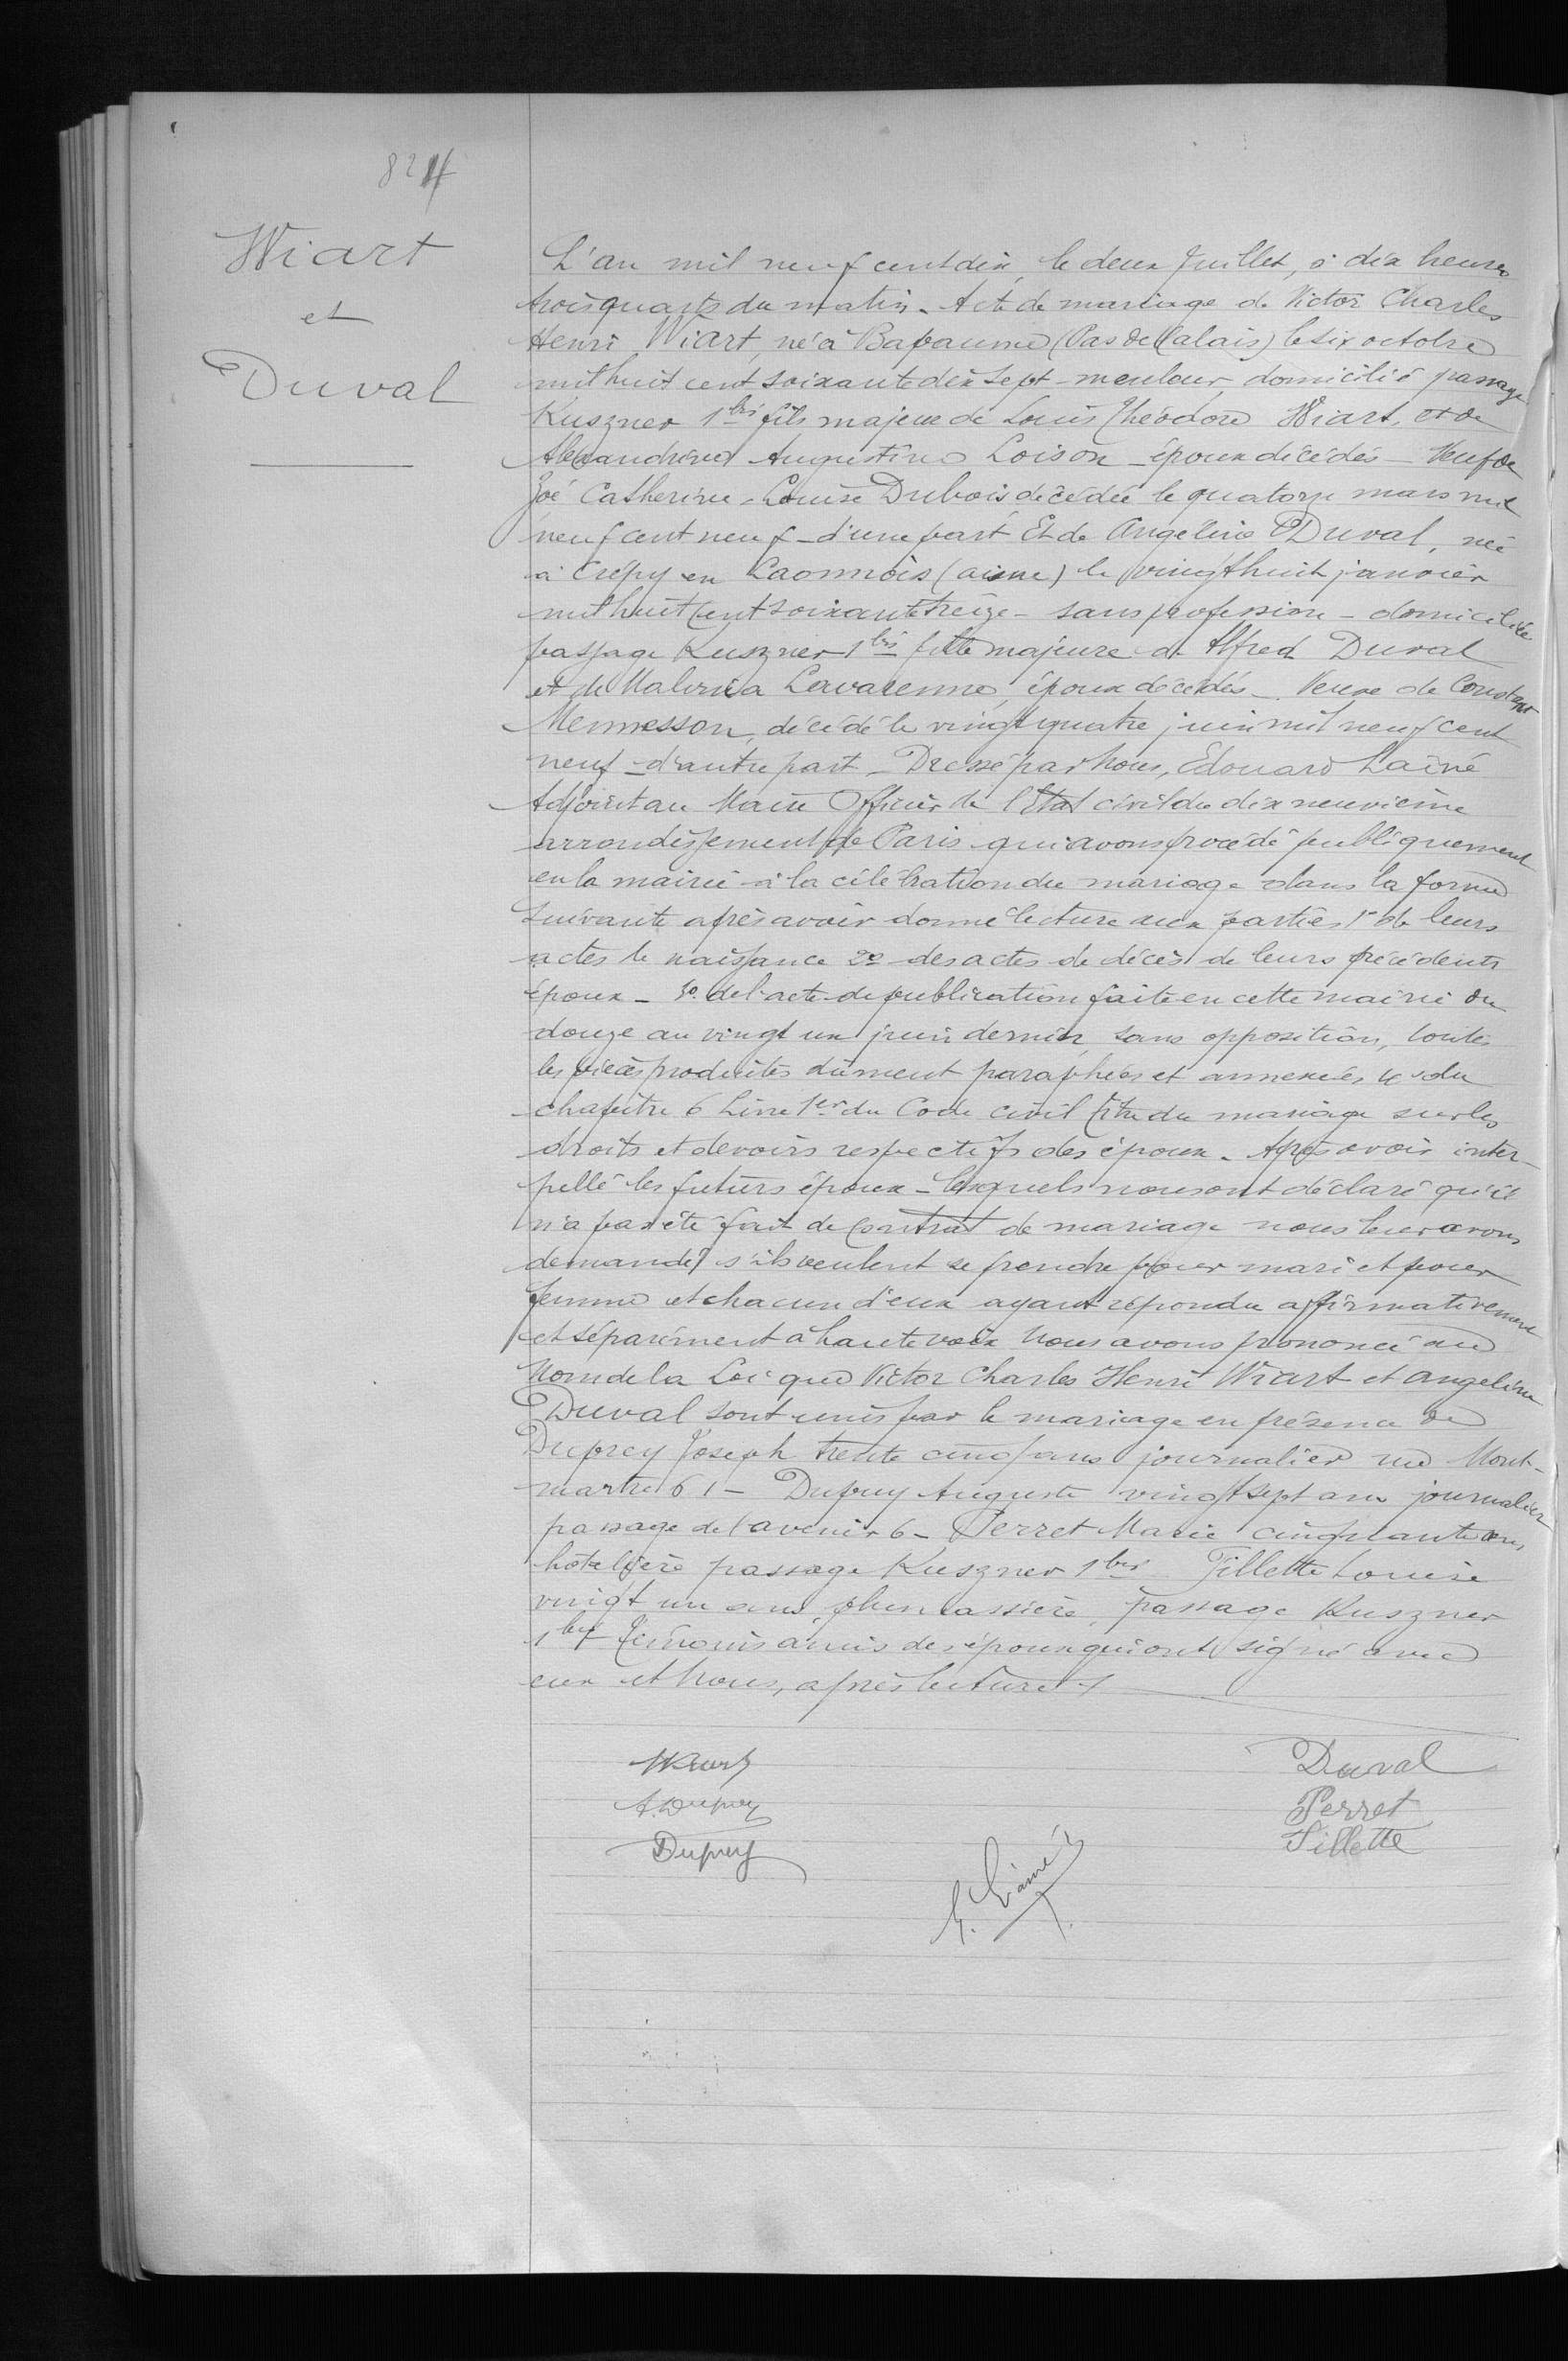824
Wiart
et
Duval
L'an mil neuf cent dix, le deux juillet à dix heures
trois quarts du matin Acte de mariage de Victor Charles
Henri, Wiart, né à Bapaume (Pas-de-Calais) le six octobre
mil huit cent soixante dix sept meuleur domicilié passage
Kuszner 1bis fils majeur de Louis Théodore Hiard et de
Alexandrine Augustino Loison époux décédés Veuf de
Zoé Catherine Louise Dubois décédée le quatorze mars mil
neuf cent neuf d'une part Et de Angélino Duval, née
à Crépy en Laomois (aisne) le vingt huit janvier
mil huit cent soixant treize sans profession domiciliée
passage Keizner 1bis fille majeure d'Alfred Duval
et de Maliria Lavarenno, époux décédés Veuve de Constar
Memesson décédé le vingt quatre juin mil neuf cent
neuf d'autre part Dressé par Nous, Edouard Lainé
Adjoint au Maire Officier de l'Etat civil du dix neuvième
arrondissement de Paris qui avons prononcé publiquement
en la mairie à la célébration du mariage dans la forme
suivante après avoir donné lecture aux parties 1° de leurs
actes de naissance 2° des actes de décès de leurs précédents
époux 3° de l'acte de publication faite en cette mairie du
douze au vingt un juin dernier sans opposition, toutes
les pièces produites dûment paraphées et annexées, 4 du
chapitre 6 Livre 1er du Code Civil Titre du mariage sur les
droits et devoirs respectifs des époux. Après avoir inter-
pellé les futurs époux lesquels nous ont déclaré qu'il
n'a pas été fait de contrat de mariage nous leur avons
demandé s'ils veulent se prendre pour mari et pour
femme et chacun d'eux ayant répondu affirmativement
et séparément à haute voix Nous avons prononcé au
Nom de la Loi que Victor Charles Henri Viart et Angelina
Duval sont unis par le mariage en présence de
Duprey Joseph trente cinq ans journalier rue Mont
martre 61-Depuy Auguste vingt sept ans journalier
passage de l'avenir 6-Perret Marie cinquante ans,
hôtelière passage Kuszner 1bis Fillette Louise
vingt un ans plumassière passage Kuszner
1 bis témoins amis de l'époux qui ont signé avec
eux et Nous, après lecture./.
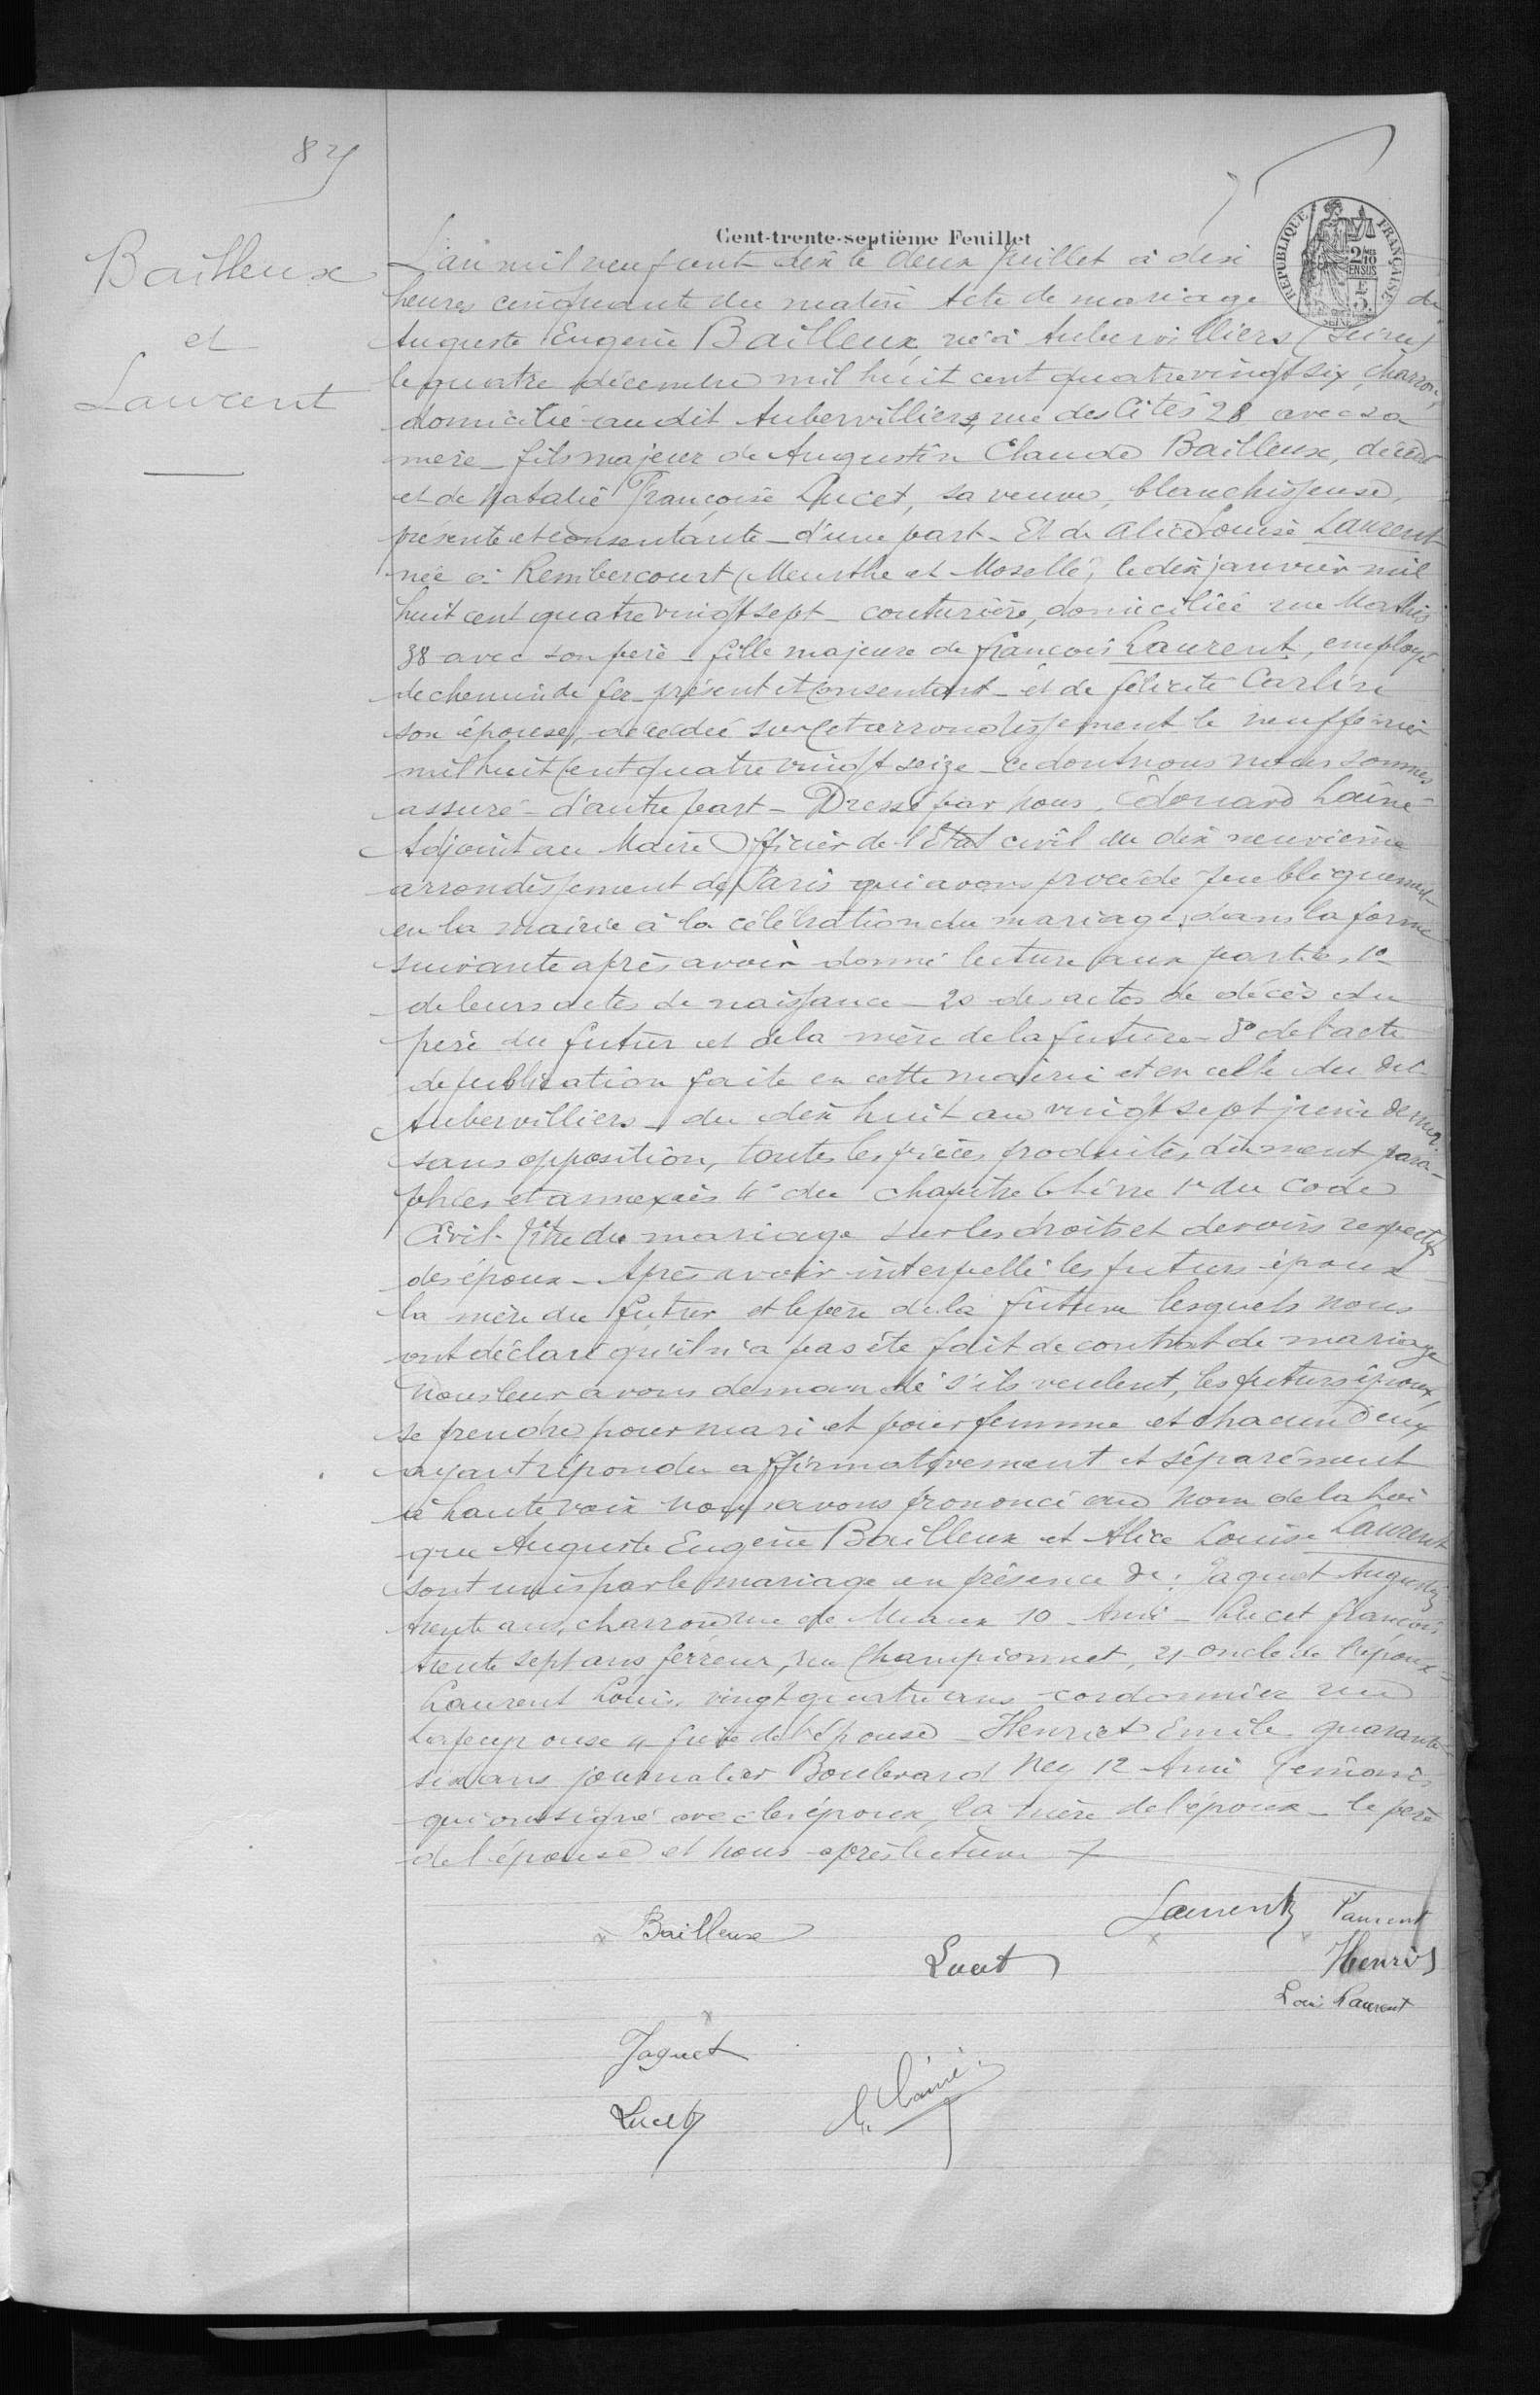84
Bailleux
et
Laurent
L'an mil neuf cent dix le deux juillet à dix
heures cinquante du matin Acte de mariage de
Auguste Eugène Bailleux, né à Aubervilliers (Seine)
le quatre décembre mil huit cent quatre vingt six, charonne
domicilié audit Aubervilliers rue des Cités 28 avec sa
mère fils majeur de Augustin Claude Bailleux décédé
et de Natalie Françoise Ducet, sa veuve, blanchisseuse,
présente et consentante -d'une part -Et de Alice Louise Laurent
née à Rembescourt (Meurthe et Moselle) le dix janvier mil
huit cent quatre vingt sept couturière, domiciliée rue Marués
38 avec son père fille majeure de françois Laurent, employé
de chemin de fer présent et consentant et de félicité Carlize
son épouse, décédée sur cet arrondissement le neuf février
mil huit cent quatre vingt seize ce dont nous nous sommes
assuré -d'autre part -Dressé par nous Edouart Laîné
Adjoint au Maire Officier de l'Etat civil du dix neuvième
arrondissement de Paris qui avons procédé publiquement
en la mairie à la célébration du mariage dans la forme
suivante après avoir donné lecture aux parties 1°
de leurs actes de naissance 2° des actes de décès du
père du futur et de la mère de la future 8° de l'acte
de publication faite en cette mairie et en celle du dit
Aubervilliers du dix huit au vingt sept juin demeure
sans opposition, toutes les pièces produites, dûment para-
phées et annexées 4° du Chapitre 6 livre 1er du Code
Civil titre du mariage sur les droits et devoirs respectifs
des époux Après avoir interpellé les futurs époux
la mère du futur et le père de la futur lesquels nous
ont déclaré qu'il n'a pas été fait de contrat de mariage
Nous leur avons demandé s'ils veulent, les futurs époux,
se prendre pour mari et pour femme et chacun d'eux
ayant répondu affirmativement et séparément
à haute voix nous avons prononcé au nom de la loi
que Auguste Eugène Bailleur et Alice Louise Laurent
sont unis par le mariage en présence de: Jaquet Augustin
trente ans, charonne, rue de Maux 10 -Ami -Lucet François
trente sept ans ferreur, rue Championnet, 21 oncle de l'époux
Laurent Louis, vingt quatre ans cordonnier rue
Lapeupouse 4, frère de l'épouse Henriet Emile quarante
six ans journalier Boulevard Ney 12 Ami Témoins
qui ont signé avec les époux la mère de l'époux le père
de l'épouse et nous après lecture./.